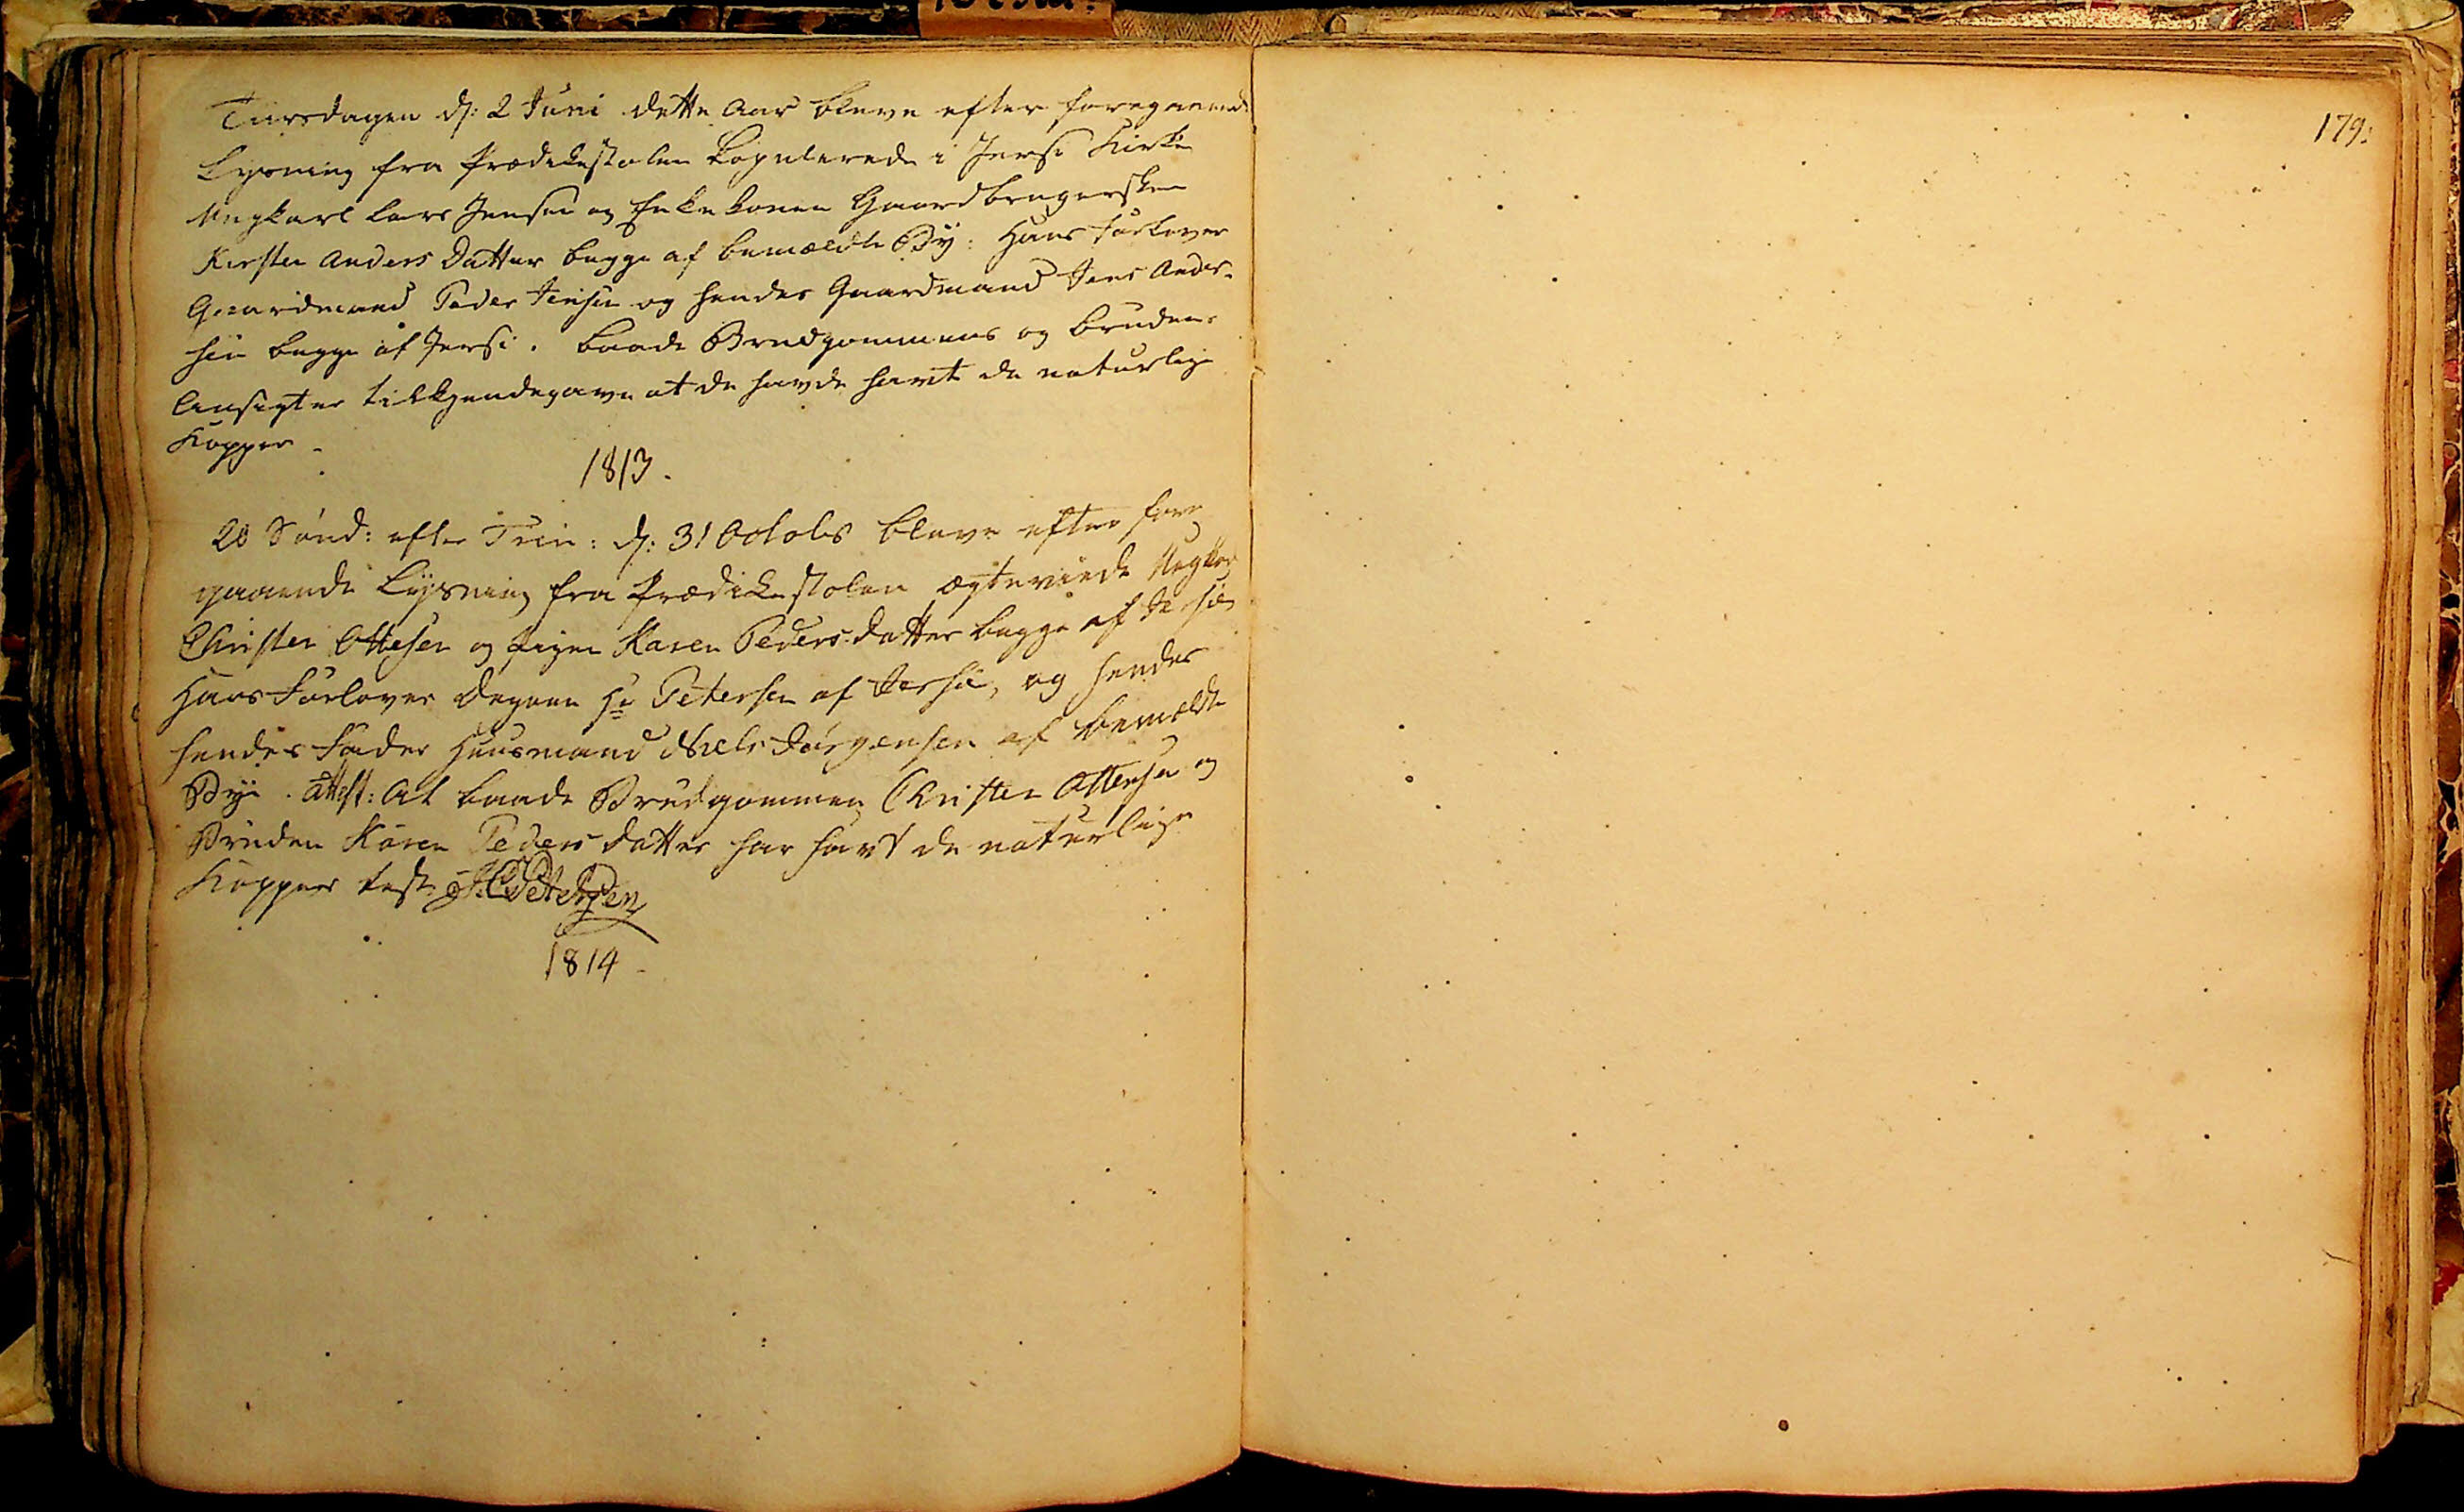Tiirsdagen d: 2 Juni dette Aar bleve efter foregaaende
Lysning fra Prædikestolen kopulerede i Jersi Kirke
Ungkarl Lars Jensen og Enkekonen Gaardbrugerske
Kirsten Anders Datter begge af bemældte By: Hans Forlover
Gaardmand Peder Jensen og hendes Gaardmand Jens Ander¬
sen begge af Jersi. Baade Brudgommens og Brudens
Ansigter tilkjendegave at de havde havt de naturlige
Kopper.
1813.
20 Sønd: efter Trin: d: 31. Octobr bleve efter fore
gaaende Lysning fra Prædikestolen ægteviede Ungkarl
Christen Ottesen og Pigen Karen Peders Datter begge af Jersie
Hans Forlover Degnen Hr Petersen af Jersie, og hendes
hendes Fader Huusmand Niels Jørgensen af bemældte
By. Attest: At baade Brudgommen Christen Ottesen og
Bruden Karen Peders Datter har havt de naturlige
Kopper test: I: C: Peterzen
1814.
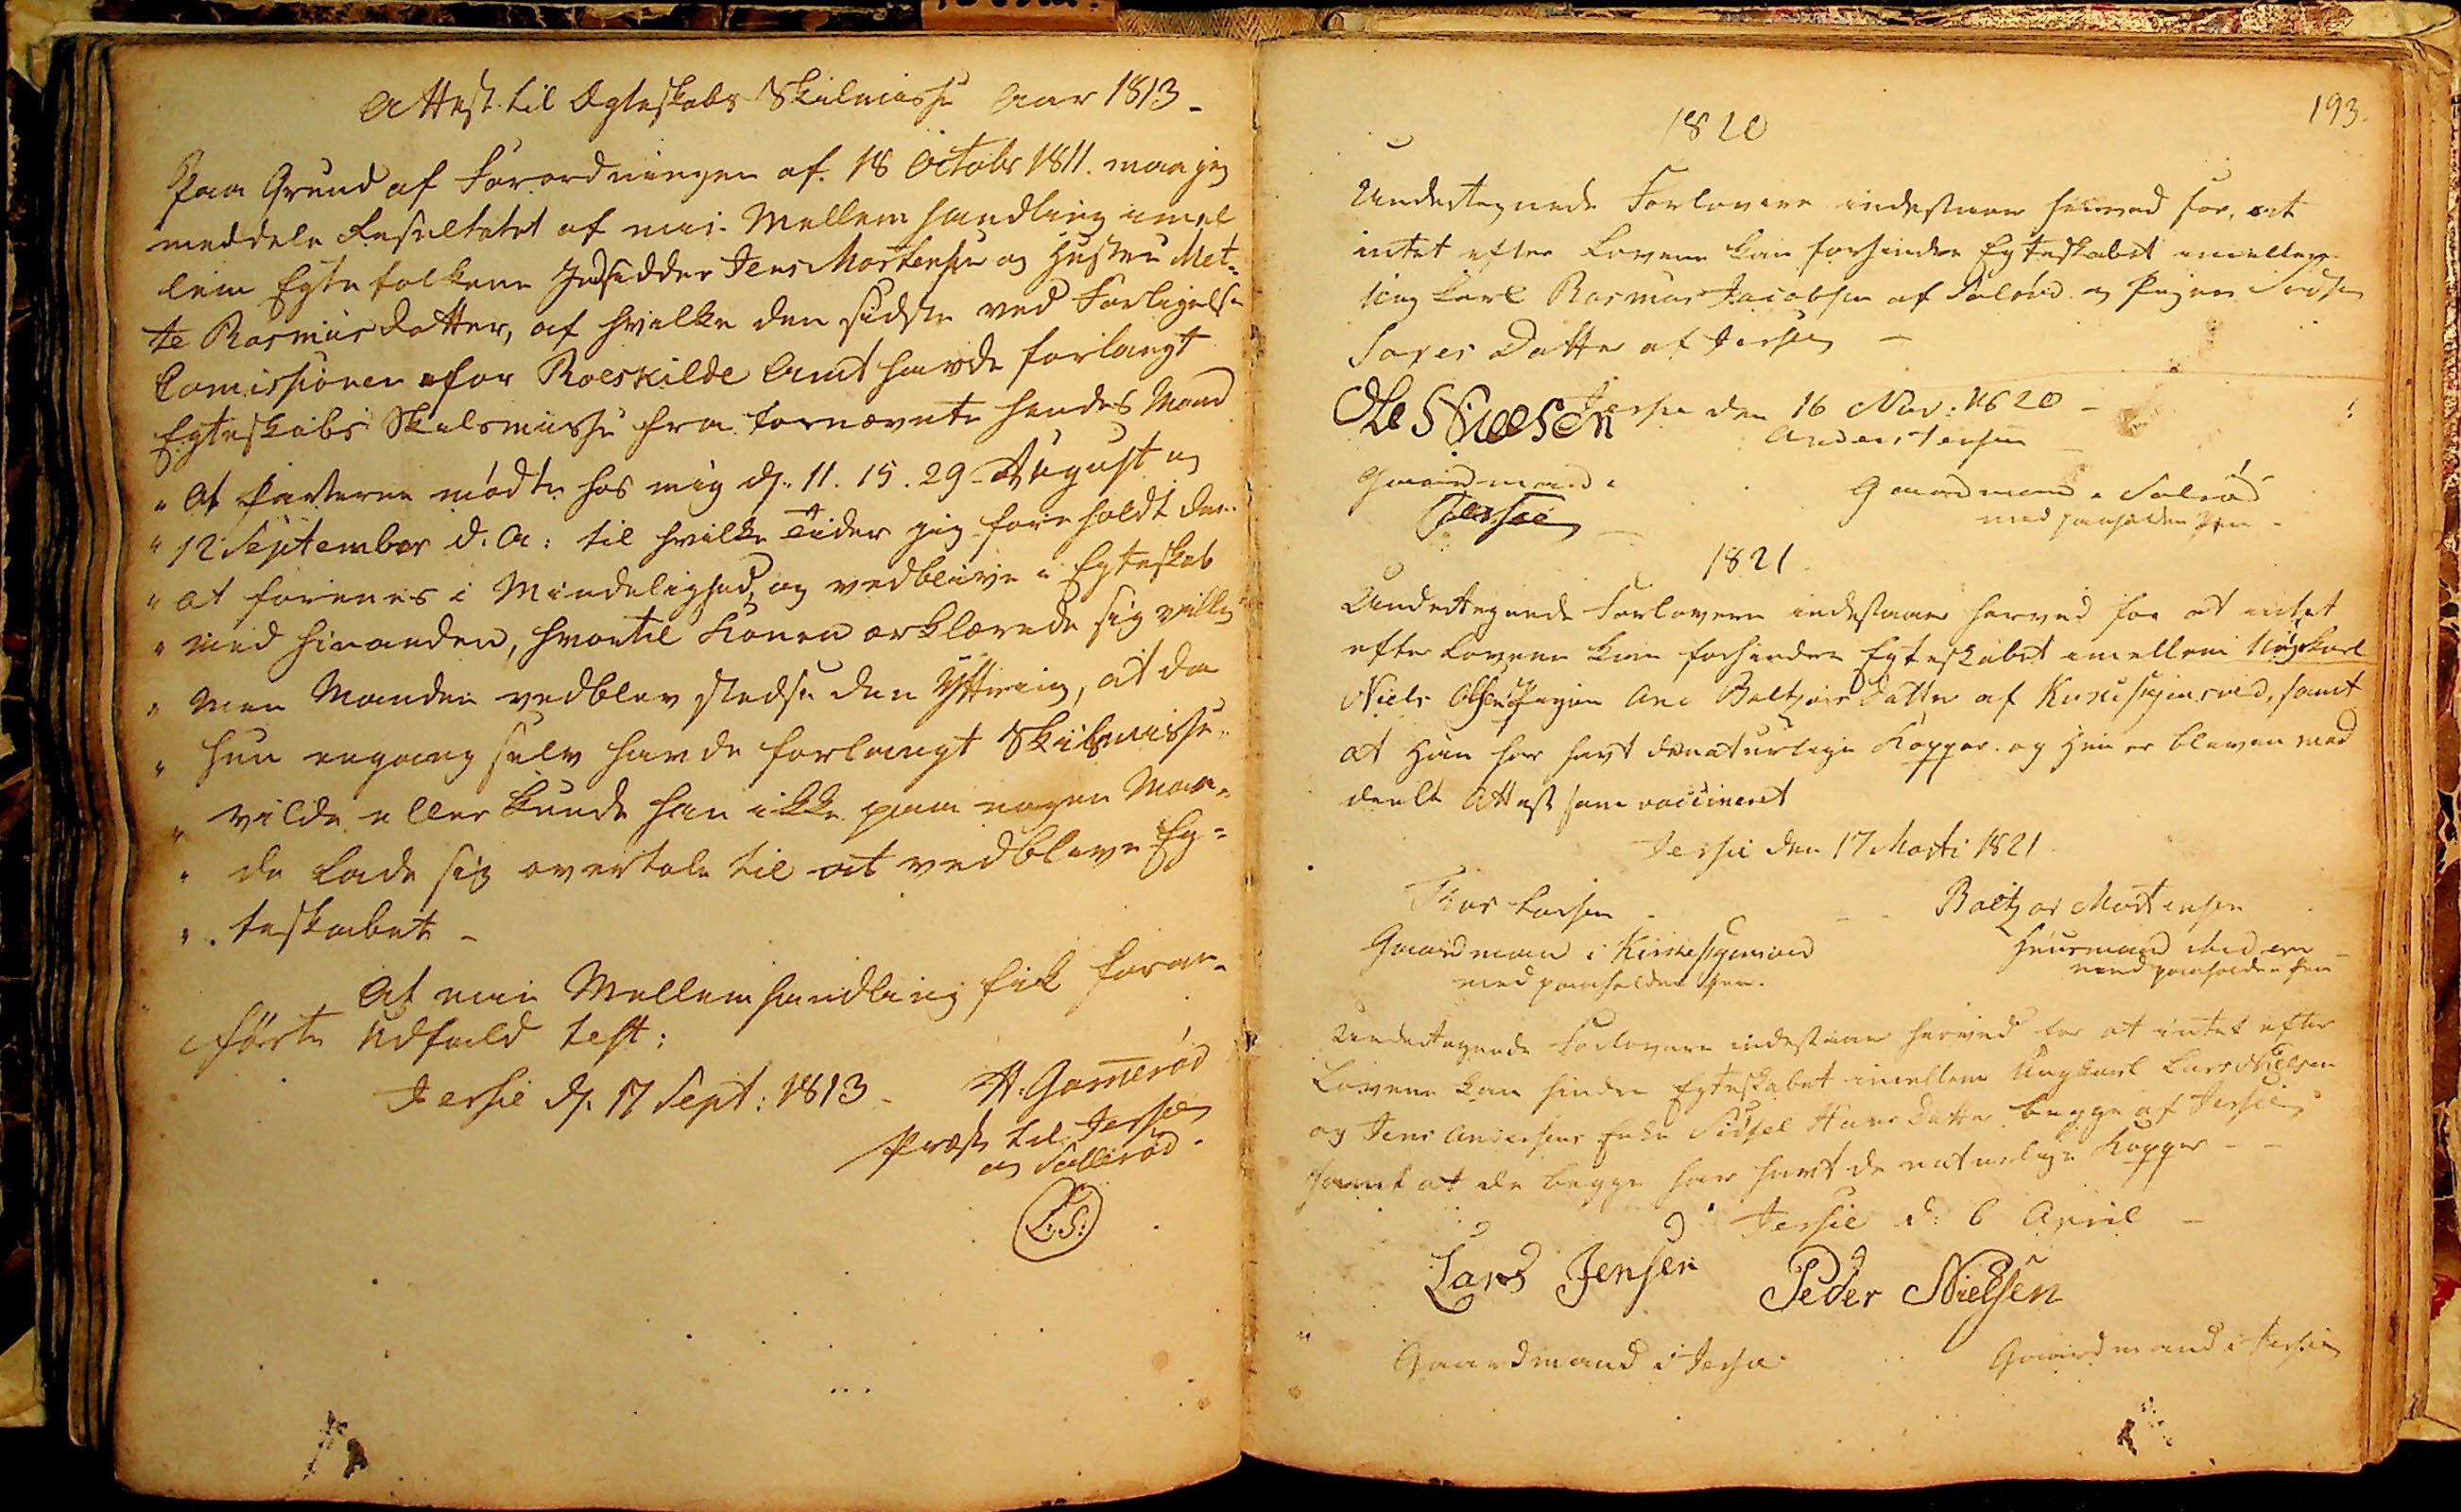Attest til Ægteskabs Skilsmisse Aar 1813.
Paa Grund af Forordningen af 18 Octobr 1811. maa jeg
meddele Resultatet af min Mellem handling imel
lem Egtefolkene Indsidder Jens Mortensen og Hustru Met¬
te Rasmusdatter, af hvilke den sidste ved Forligelse
Comissionen for Roeskilde Amt havde forlangt
Egteskabs Skilsmisse fra fornævnte hendes Mand
At Parterne mødte hos mig d. 11. 15. 29. August og
12 September d: A: til hvilke Tider jeg foreholdt dem
at forenes i Mindelighed, og vedblive i Egteskab
med hinanden, hvortil Konen erklærede sig villig
men Manden vedblev stedse den Yttring, at da
hun engang selv havde forlangt Skilsmisse
vilde eller kunde han ikke paa nogen Maa¬
de lade sig overtale til at vedblive Eg¬
teskabet 
At min Mellemhandling fik foran¬
førte Udfald test:
Jersie d: 17 Sept: 1813.   
H: Gamerød
Præst til Jersie
L:S:
og Sollerød.
193.
1820
Undertegnede Forlovere indestaar herved for, at
intet efter Lovene kan forhindre Egteskabet imellem
Ung karl Rasmus Jacobsen af Solrød og Pigen Sidse
Saxes Datter af Jersie
Jersie den 16 Nov: 1820 -
Ole Nielsen   
Anders Jensen
Gaardmand i        
Gaardmand i Solrød
Jersie           
med paaholden Pen
1821
Undertegnede Forlovere indestaar herved for at intet
efter Lovene kan forhindre Egteskabet imellem Ungkarl
Niels Olsen og Pigen Ane Baltzar Datter af Kirkeskjensved, samt
at Han har havt de naturlige Kopper og Hun er bleven med
deelt Attest som vaccineret
Jersie den 17 Marti 1821
Thor Larsen             
Baltzar Mortensen
Gaardmand i Kirkeskjensved         
Huusmand ibidem
med paaholden Pen.             
med paaholden Pen.
Undertegnede Forlovere indestaar herved for at intet efter
Lovene kan hindre Egteskabet imellem Ungkarl Lars Nielsen
og Jens Andersens Enke Sidsel Hans Datter begge af Jersie
samt at de begge har havt de naturlige Kopper
Jersie d: 6 April
Lars Jensen
Peder Nielsen
Gaardmand i Jersie
Gaardmand i Jersie